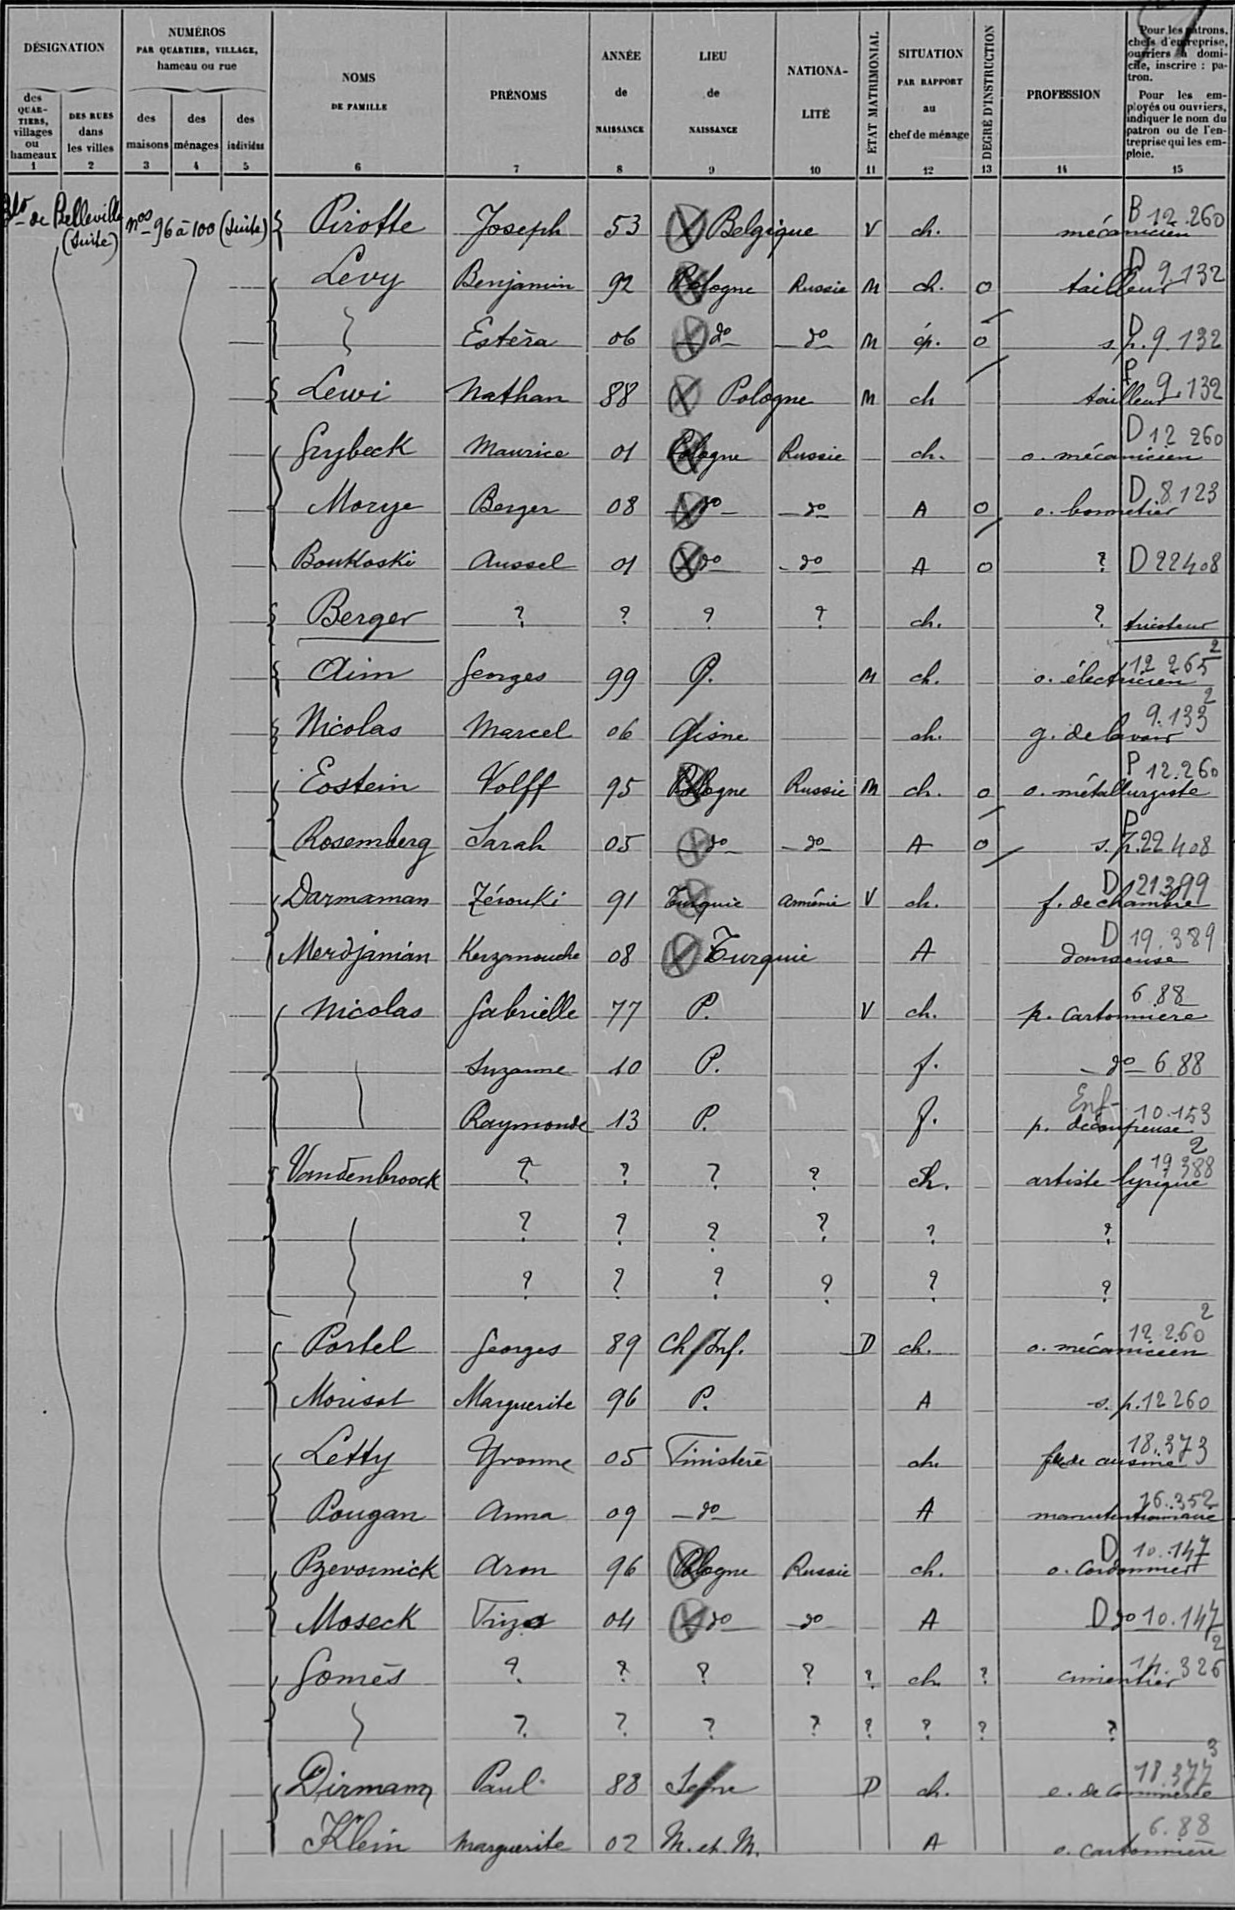Pirotte/Joseph/53/Belgique/¤/V/ch /¤/mécanicien/B
Levy/Benjamin/92/Pologne/Russie/M/ch /0/tailleur/¤
¤/Estèra/06/d°/d°/M/ép /0/s p /9132
Lewi/Nathan/88/Pologne/¤/M/ch/¤/tailleur/9132
Grybeck/Maurice/01/Pologne/Russie/¤/ch /¤/o mécanicien/¤
Morye/Berger/08/d°/d°/¤/A/0/o bonnetier/¤
Boukoski/Anssel/01/d°/d°/¤/A/0/¤/D 22408
Berger/¤/¤/¤/¤/¤/ch /¤/¤/tricoteur
Aim/Georges/99/P /¤/M/ch /¤/o électricien/¤
Nicolas/Marcel/06/Aisne/¤/¤/ch /¤/g de lavoir/¤
Eostein/Volff/95/Pologne/Russie/M/ch /0/o métallurgiste/¤
Rosemberg/Sarah/05/d°/d°/¤/A/0/s p /22408
Darmaman/Zérouki/91/Turquie/arménie/V/ch /¤/f de chambre/¤
Merdjamian/Kerzanouche/08/Turquie/¤/¤/A/¤/danseuse/¤
Nicolas/Gabrielle/77/P /¤/V/ch /¤/p cartonnière/¤
¤/Suzanne/10/P /¤/¤/f /¤/d°/688
¤/Raymonde/13/P /¤/¤/f /¤/p découpeuse/¤
Vandenbroock/¤/¤/¤/¤/¤/ch /¤/artiste lyrique/¤
¤/¤/¤/¤/¤/¤/¤/¤/¤/¤
¤/¤/¤/¤/¤/¤/¤/¤/¤/¤
Portel/Georges/89/Ch:Inf /¤/D/ch /¤/o mécanicien/¤
Morisot/Marguerite/96/P /¤/¤/A/¤/s p /12260
Letty/Yvonne/05/Finistère/¤/¤/ch /¤/fille de cuisine/¤
Pougan/Anna/09/d°/¤/¤/A/¤/manutentionnaire/¤
Pzevornick/Aron/96/Pologne/Russie/¤/ch /¤/o cordonnier/¤
Moseck/Friza/04/d°/d°/¤/A/¤/D d°/10147
Gomès/¤/¤/¤/¤/¤/ch/¤/cimentier/¤
¤/¤/¤/¤/¤/¤/¤/¤/¤/¤
Dirmann/Paul/88/Seine/¤/D/ch /¤/e de commerce/¤
Klein/Marguerite/02/M et M /¤/¤/A/¤/o cartonnière/¤
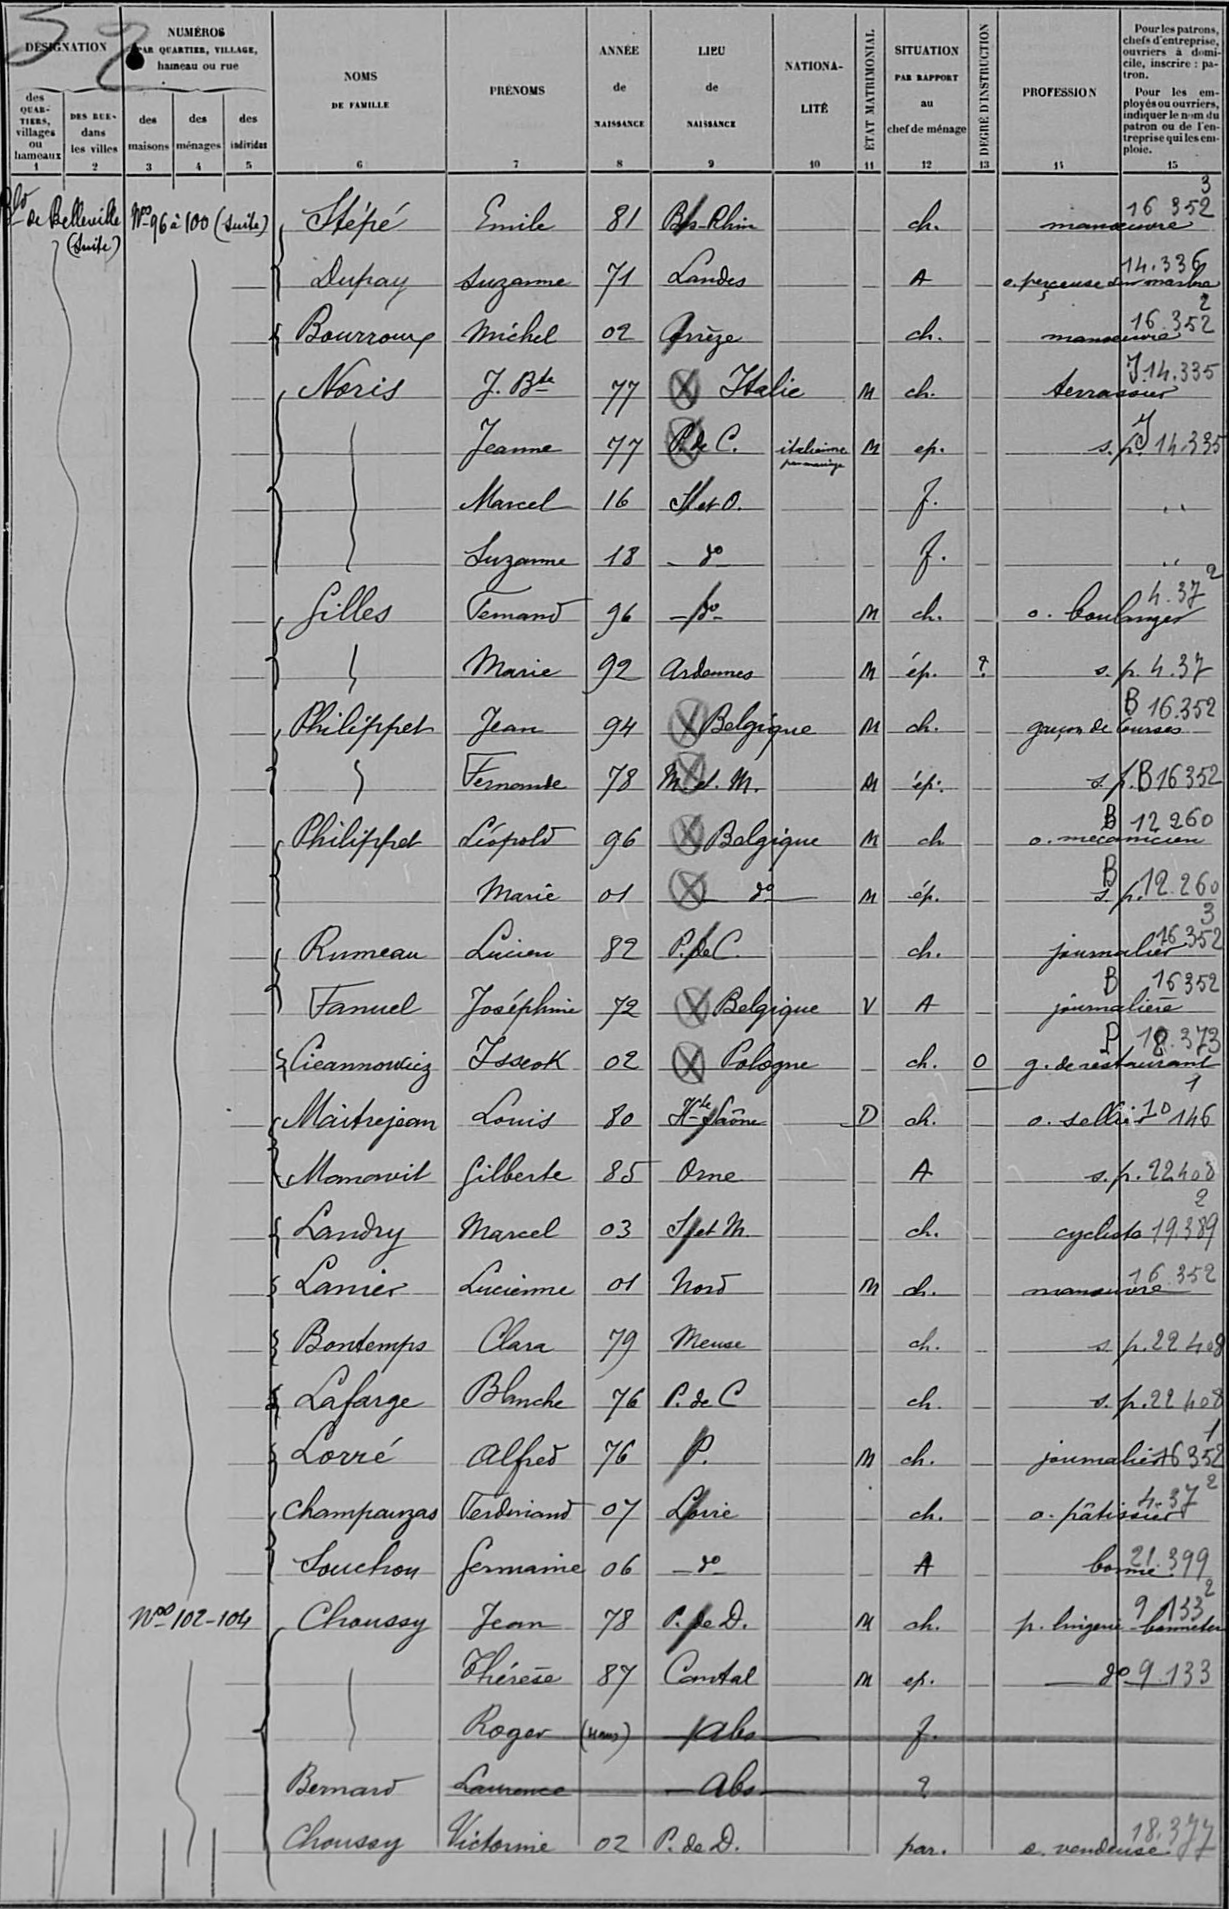Stépé/Emile/81/Bas-Rhin/¤/¤/ch /¤/manoeuvre/¤
Dupay/Suzanne/71/Landes/¤/¤/A/¤/o perçeuse sur marbre/¤
Bourroux/Michel/02/Corrèze/¤/¤/ch /¤/manoeuvre/¤
Noris/J Bte/77/Italie/¤/M/ch /¤/terrassier/¤
¤/Jeanne/77/P de C /italienne/M/ep /¤/s p /J14335
¤/Marcel/16/S et O /¤/¤/f /¤/¤/¤
¤/Suzanne/18/d°/¤/¤/f /¤/¤/¤
Gilles/Fernand/96/d°/¤/M/ch /¤/o boulanger/¤
¤/Marie/92/Ardennes/¤/M/ép /¤/s p /437
Philippet/Jean/94/Belgique/¤/M/ch /¤/garçon de courses/¤
¤/Fernande/78/M et M /¤/M/ép /¤/s p /B16352
Philippet/Léopold/96/Belgique/¤/M/ch/¤/o mécanicien/¤
¤/Marie/01/d°/¤/M/ép /¤/¤/s p /12260
Rumeau/Lucien/82/P de C /¤/¤/ch /¤/journalier/16352
Fanuel/Jospéphine/72/Belgique/¤/V/A/¤/journalière/¤
Cieannowicz/Isscok/02/Pologne/¤/¤/ch /0/g de restaurant/¤
Maitrejean/Louis/80/Hte Saône/¤/D/ch /¤/o sellier/10146
Momovit/Gilberte/85/Orne/¤/¤/A/¤/s p /22408
Landry/Marcel/03/S et M/¤/¤/ch /¤/cycliste/19389
Lanier/Lucienne/01/Nord/¤/M/ch /¤/manoeuvre/¤
Bontemps/Clara/79/Meuse/¤/¤/ch /¤/s p /22408
Lafarge/Blanche/76/P de C/¤/¤/ch /¤/s p /22408
Lorré/Alfred/76/P /¤/M/ch /¤/journalier/16352
Champauzas/Ferdinand/07/Loire/¤/¤/ch /¤/o pâtissier/¤
Souchon/Gemaine/06/d°/¤/¤/A/¤/bonne/21399
Choussy/Jean/78/P de D /¤/M/ch /¤/p lingerie-bonneterie/¤
¤/Thérèse/87/Cantal/¤/M/ep /¤/d°/9133
¤/Roger/(4 ans)/-abs-/¤/¤/f /¤/¤/¤
Bernard/Laurence/¤/-abs-/¤/¤/¤/¤/¤/¤
Choussy/Victorine/02/P de D /¤/¤/par /¤/e vendeuse/¤